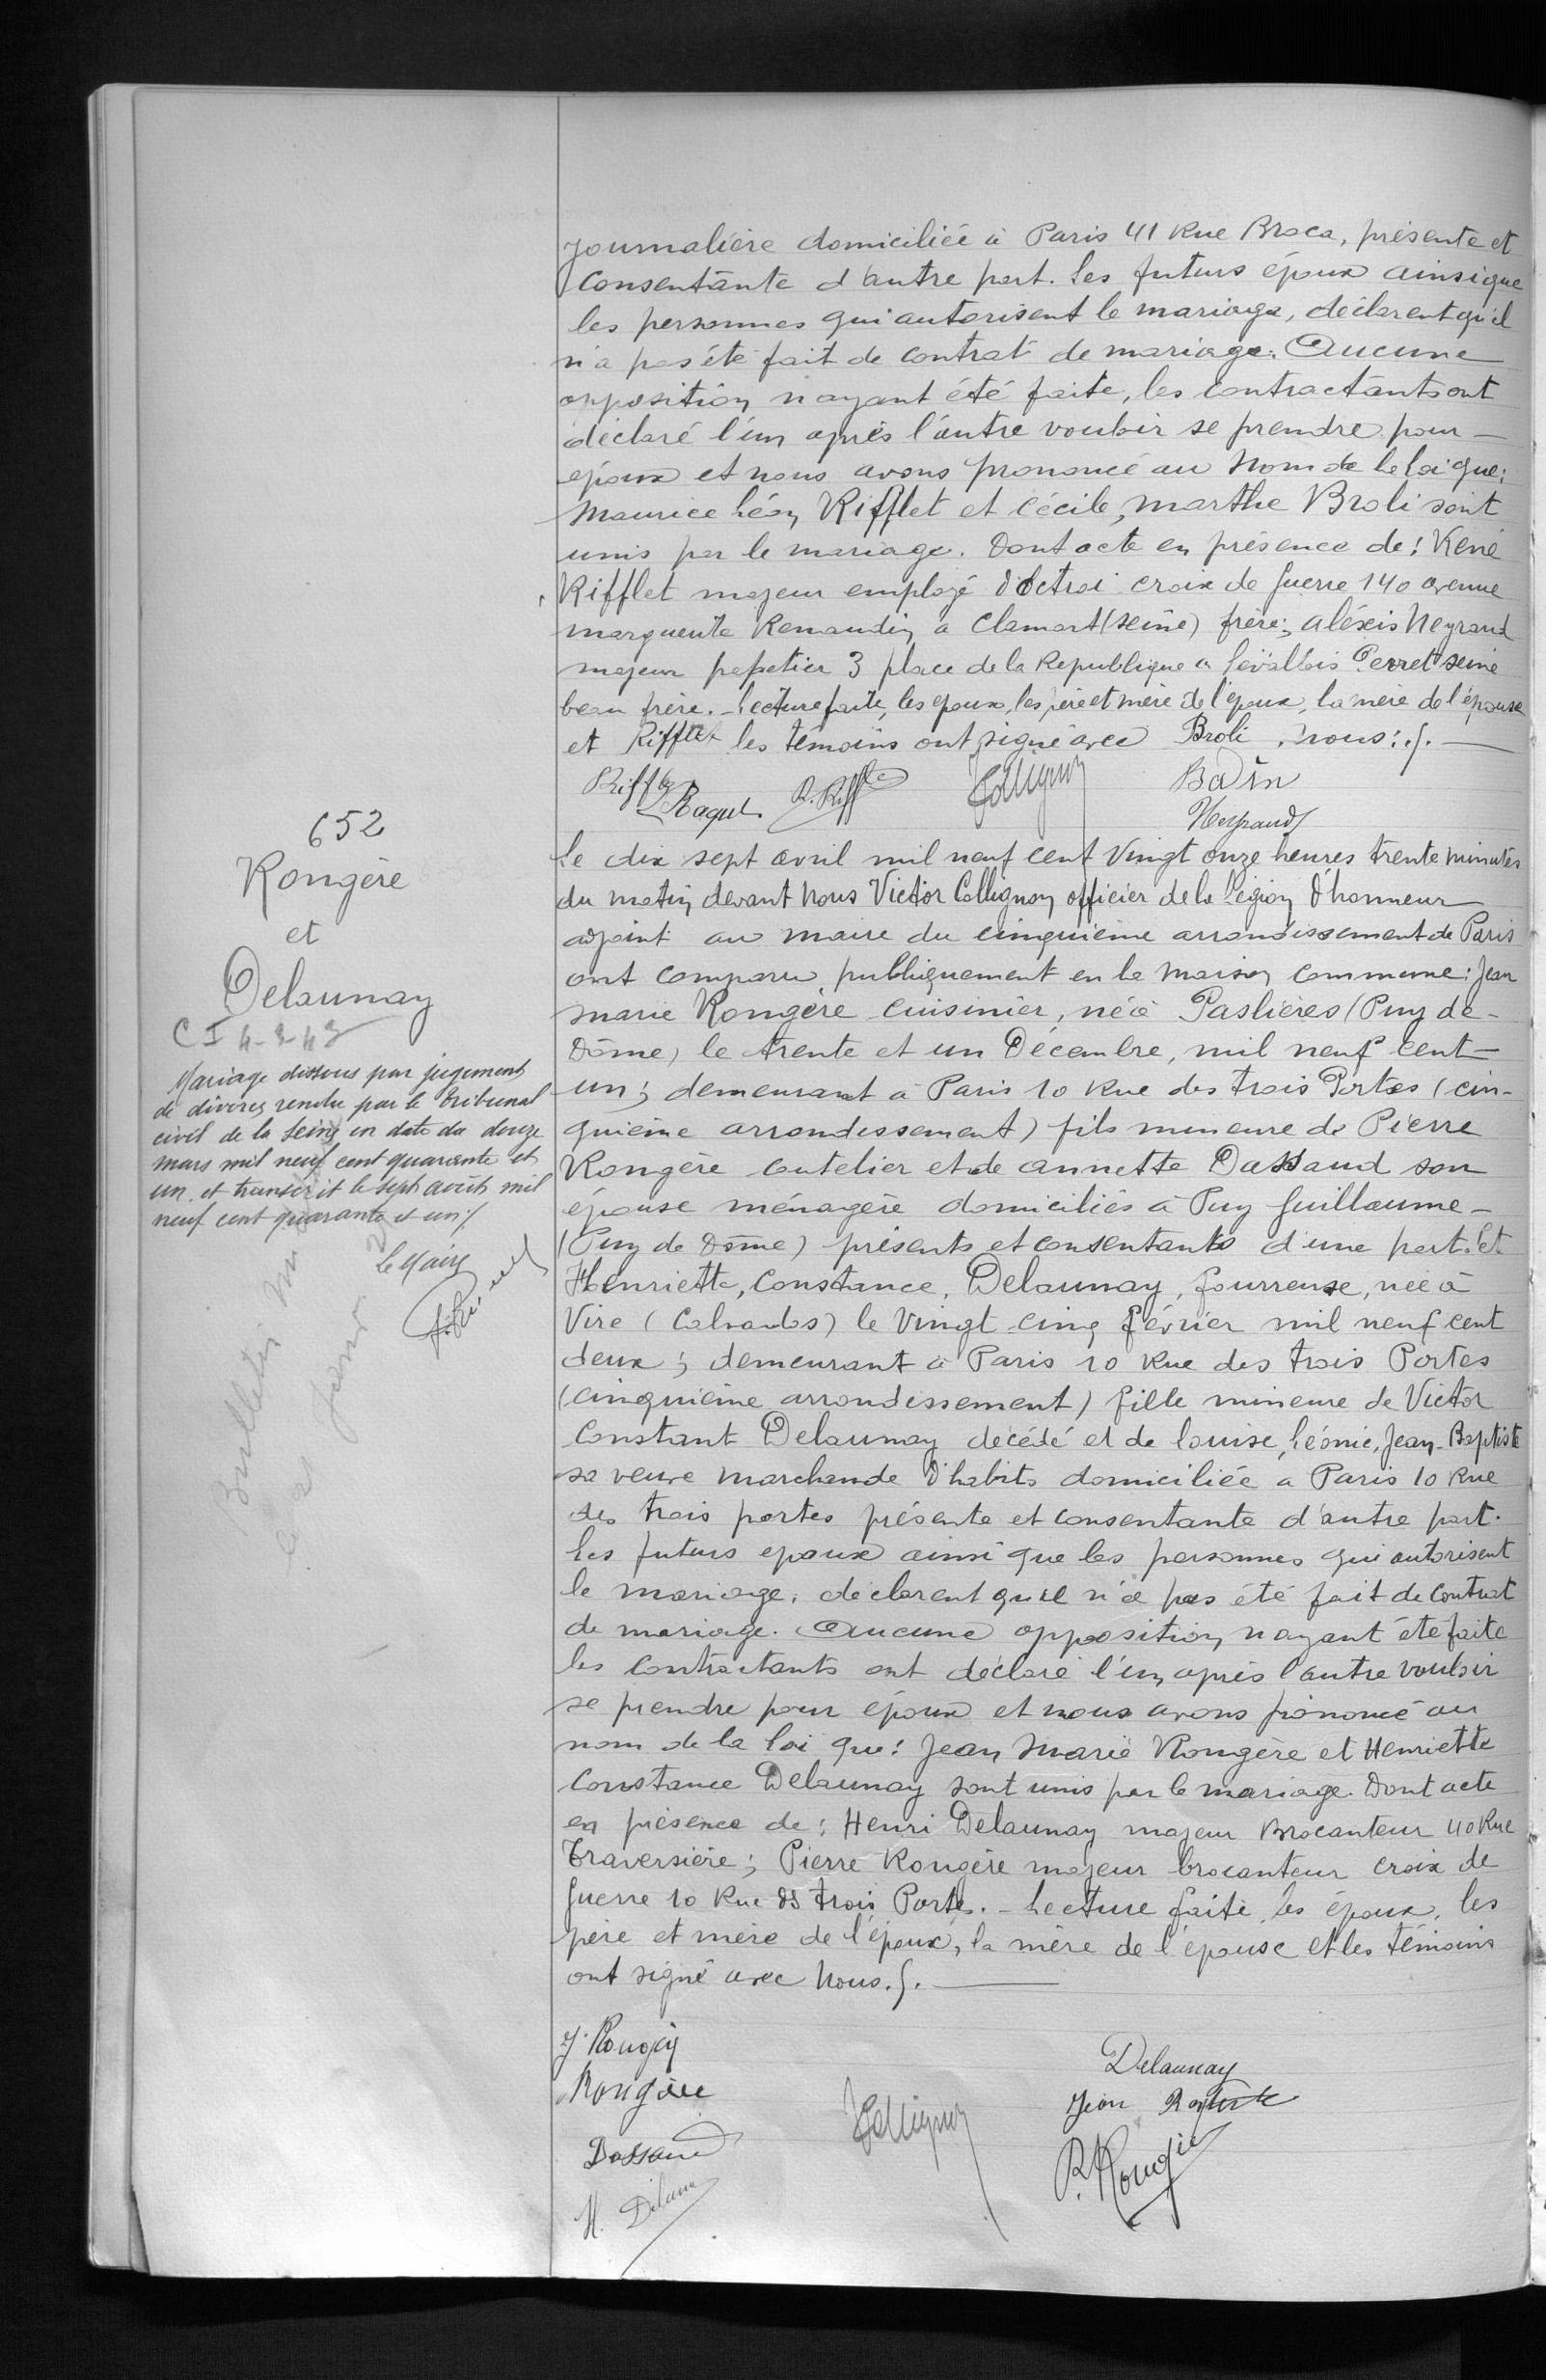journalière domiciliée à Paris 41 rue Broca, présente et
consentante d'autre part. Les futurs époux ainsi que
les personnes qui autorisent le mariage déclarent qu'il
n'a pas été fait de contrat de mariage. Aucune
opposition n'ayant été faite, les contractants ont
déclaré l'un après l'autre vouloir se prendre pour
époux et nous avons prononcé au Nom de la loi que;
Maucie Héon, Rifflet et Cécile, Marthe Broli sont
unis par le mariage. Dont acte en présence de: René
Rifflet majeur employé d'octroi croix de guerre 140 avenue
marguerite Romandin, à Clamart (Seine) frère; Aléxis Neyrand
majeur papetier 3 place de la Republique a Levallois Perret seine
beau père.-Lecture faite les époux, les père et mère de l'époux, la mère de l'épouse
et Rifflet, les témoins ont signé avec Broli nous ./.
652
Rongère
et
Delaunay
I4-3-43
Le dix sept avril mil neuf cent vingt onze heures trente minutes
du matin, devant Nous Victor Collignon, officier de la légion d'honneur
adjoint au maire du cinquième arrondissement de Paris
ont comparu publiquement en la maison commune: Jean
Marie Rongère Cuisinier, née à Paslières (Puy de
Dôme) le trente et un Décembre, mil neuf cent-
un; demeurant à Paris 10 Rue des trois Portes (cin-
quième arrondissement) fils mineure de Pierre
Rongère coutelier et de annette dassaud son
épouse ménagère domiciliés à Puy Guillaume-
(Puy de Dôme) présents et consentants d'une part. et
Henriette, Constance, Delaumay, fourreuse, née à
Vire (Calvados) le Vingt-cinq février mil neuf cent
deux; demeurant à Paris 10 Rue des trois Portes
(cinquième arrondissement) fille mineure de Victor
Constant Delaumay décédé et de louise, léonie, Jean-Baptiste
sa veuve marchande d'habits domiciliée à Paris 10 Rue
des trois portes présente et consentante d'autre part.
les futurs époux ainsi que les personnes qui autorisent
le mariage, déclarent qu'il n'a pas été fait de contrat
de mariage. Aucune opposition, n'ayant été faite
les contractants ont déclaré l'un après l'autre vouloir
se prendre pour époux et nous avons prononcé au
nom de la loi qui: Jean Marie Rougère et Henriette
Constance Delaunay sont unis par le mariage. Dont acte
en présence de: Henri Delaunay majeur Brocanteur 40 Rue
Traversière; Pierre Rougère majeur brocanteur Croix de
fuerre 10 rue des trois Portes .-Lecture faite les époux les
père et mère de l'époux, la mère de l'épouse et les témoins
ont signé avec Nous./.-
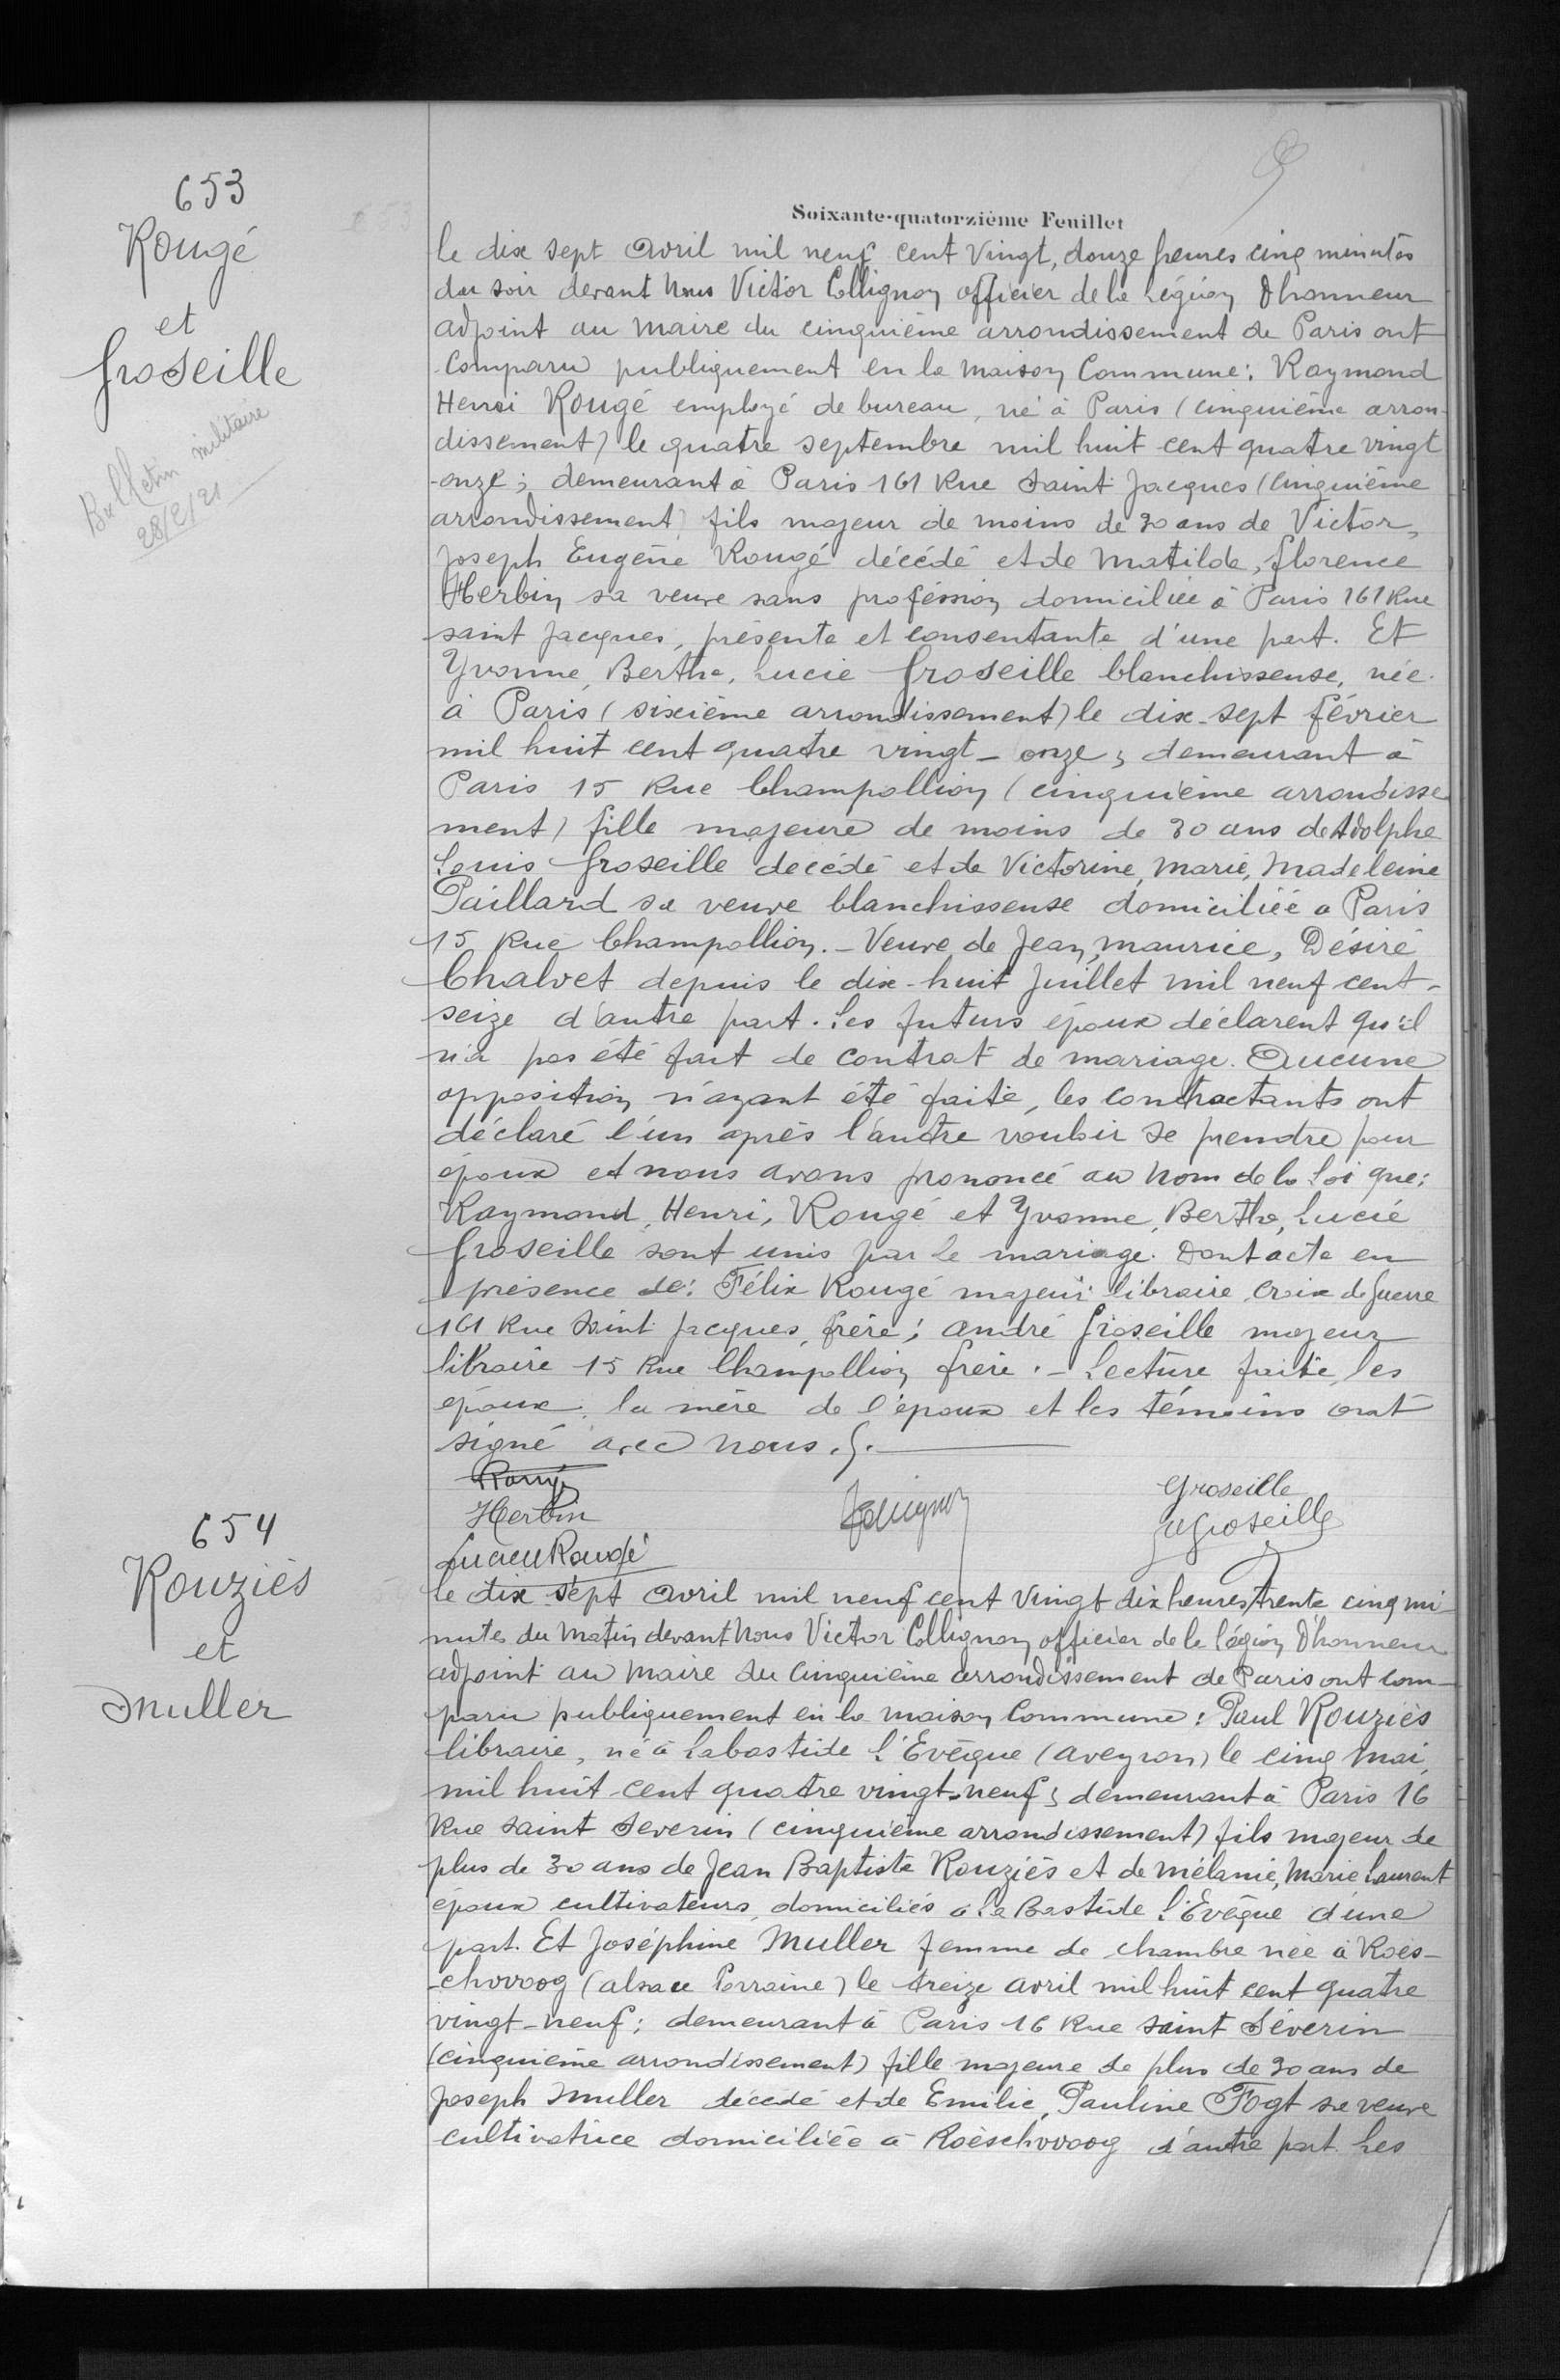653
Rougé
et
Groseille
le dix sept Avril mil neuf cent vingt, douze heures cinq minutes
du soir devant Nous Victor Collignon officier de la légion d'honneur
adjoint au maire du cinquième arrondissement de Paris ont
Comparu publiquement en la maison Commune: Raymond
Henri Rougé employé de bureau, né à Paris (Cinquième arron-
dissement) le quatre septembre mil huit cent quatre vingt
-onze; demeurant à Paris 161 Rue Saint Jacques (Cinquième
arrondissement) fils majeur de moins de 30 ans de Victor
Joseph Eugène Rougé décédé et de Matilde Florence
Herbin, sa veuve sans profession, domiciliée à Paris 161 Rue
saint Jacques, présente et consentante d'une part. Et
Yvonne Berthe, lucie Groseille blanchisseuse, née
à Paris (sixième arrondissement) le dix-sept février
mil huit cent quatre vingt-onze, demeurant à
Paris 15 Rue Champollion (cinquième arrondisse
ment) fille majeure de moins de 30 ans de Adolphe
Louis Groseille décédé et de Victorine Marie Madeleine
Paillard sa veuve blanchisseuse domiciliée à Paris
15 Rue Champollion .-Veuve de Jean, Maurice, Désiré
Chalvet depuis le dix-huit Juillet mil neuf cent-
seize d'autre part. les futurs époux déclarent qu'il
n'a pas été fait de contrat de mariage. Aucune
opposition n'ayant été faite, les contractants ont
déclaré l'un après l'autre vouloir se prendre pour
époux et nous avons prononcé au nom de la loi que:
Raymond, Henri, Rougé et Yvonne, Berthe, Lucie
Groseille sont unis par le mariage. Dont acte en
présence de: Félix Rougé majeur, libraire Croix de Guerre
161 Rue Saint Jacques, frère, André Groseille majeur
libraire 15 Rue Champollion; frère .-lecture faite les
époux, la mère de l'époux et les témoins ont
signé avec nous./.
654
Rouziès
et
Onuller
Le dix sept Avril mil neuf cent Vingt dix heures trente cinq mi
nute du Matin devant Nous Victor Collignon, officier de la légion d'honneur
adjoint au Maire du cinquième arrondissement de Paris ont com
paru publiquement en la maison Commune: Paul Rouziès
libraire né à la bastide l'Evèque (aveyron) le cinq mai
mil huit cent quatre vingt-neufs demeurant à Paris 16
Rue Saint Severin (cinquième arrondissement) fils majeur de
plus de 30 ans de Jean Baptiste Rouziès et de Mélanie, Marie Lament
époux cultivateurs, domiciliés à la Bastide l'Evêque d'une
part. Et Joséphine Muller femme de chambre née à Roes-
chvroy (alsace lorraine) le treize avril mil huit cent quatre
vingt-neuf; demeurant à Paris 16 rue Saint Séverin
(cinquième arrondissement) fille majeure de plus de 20 ans de
Joseph Muller décédé et de Emilie, Pauline Fogt sa veuve
cultivatrice domiciliée à Roèschvroy, d'autre part les